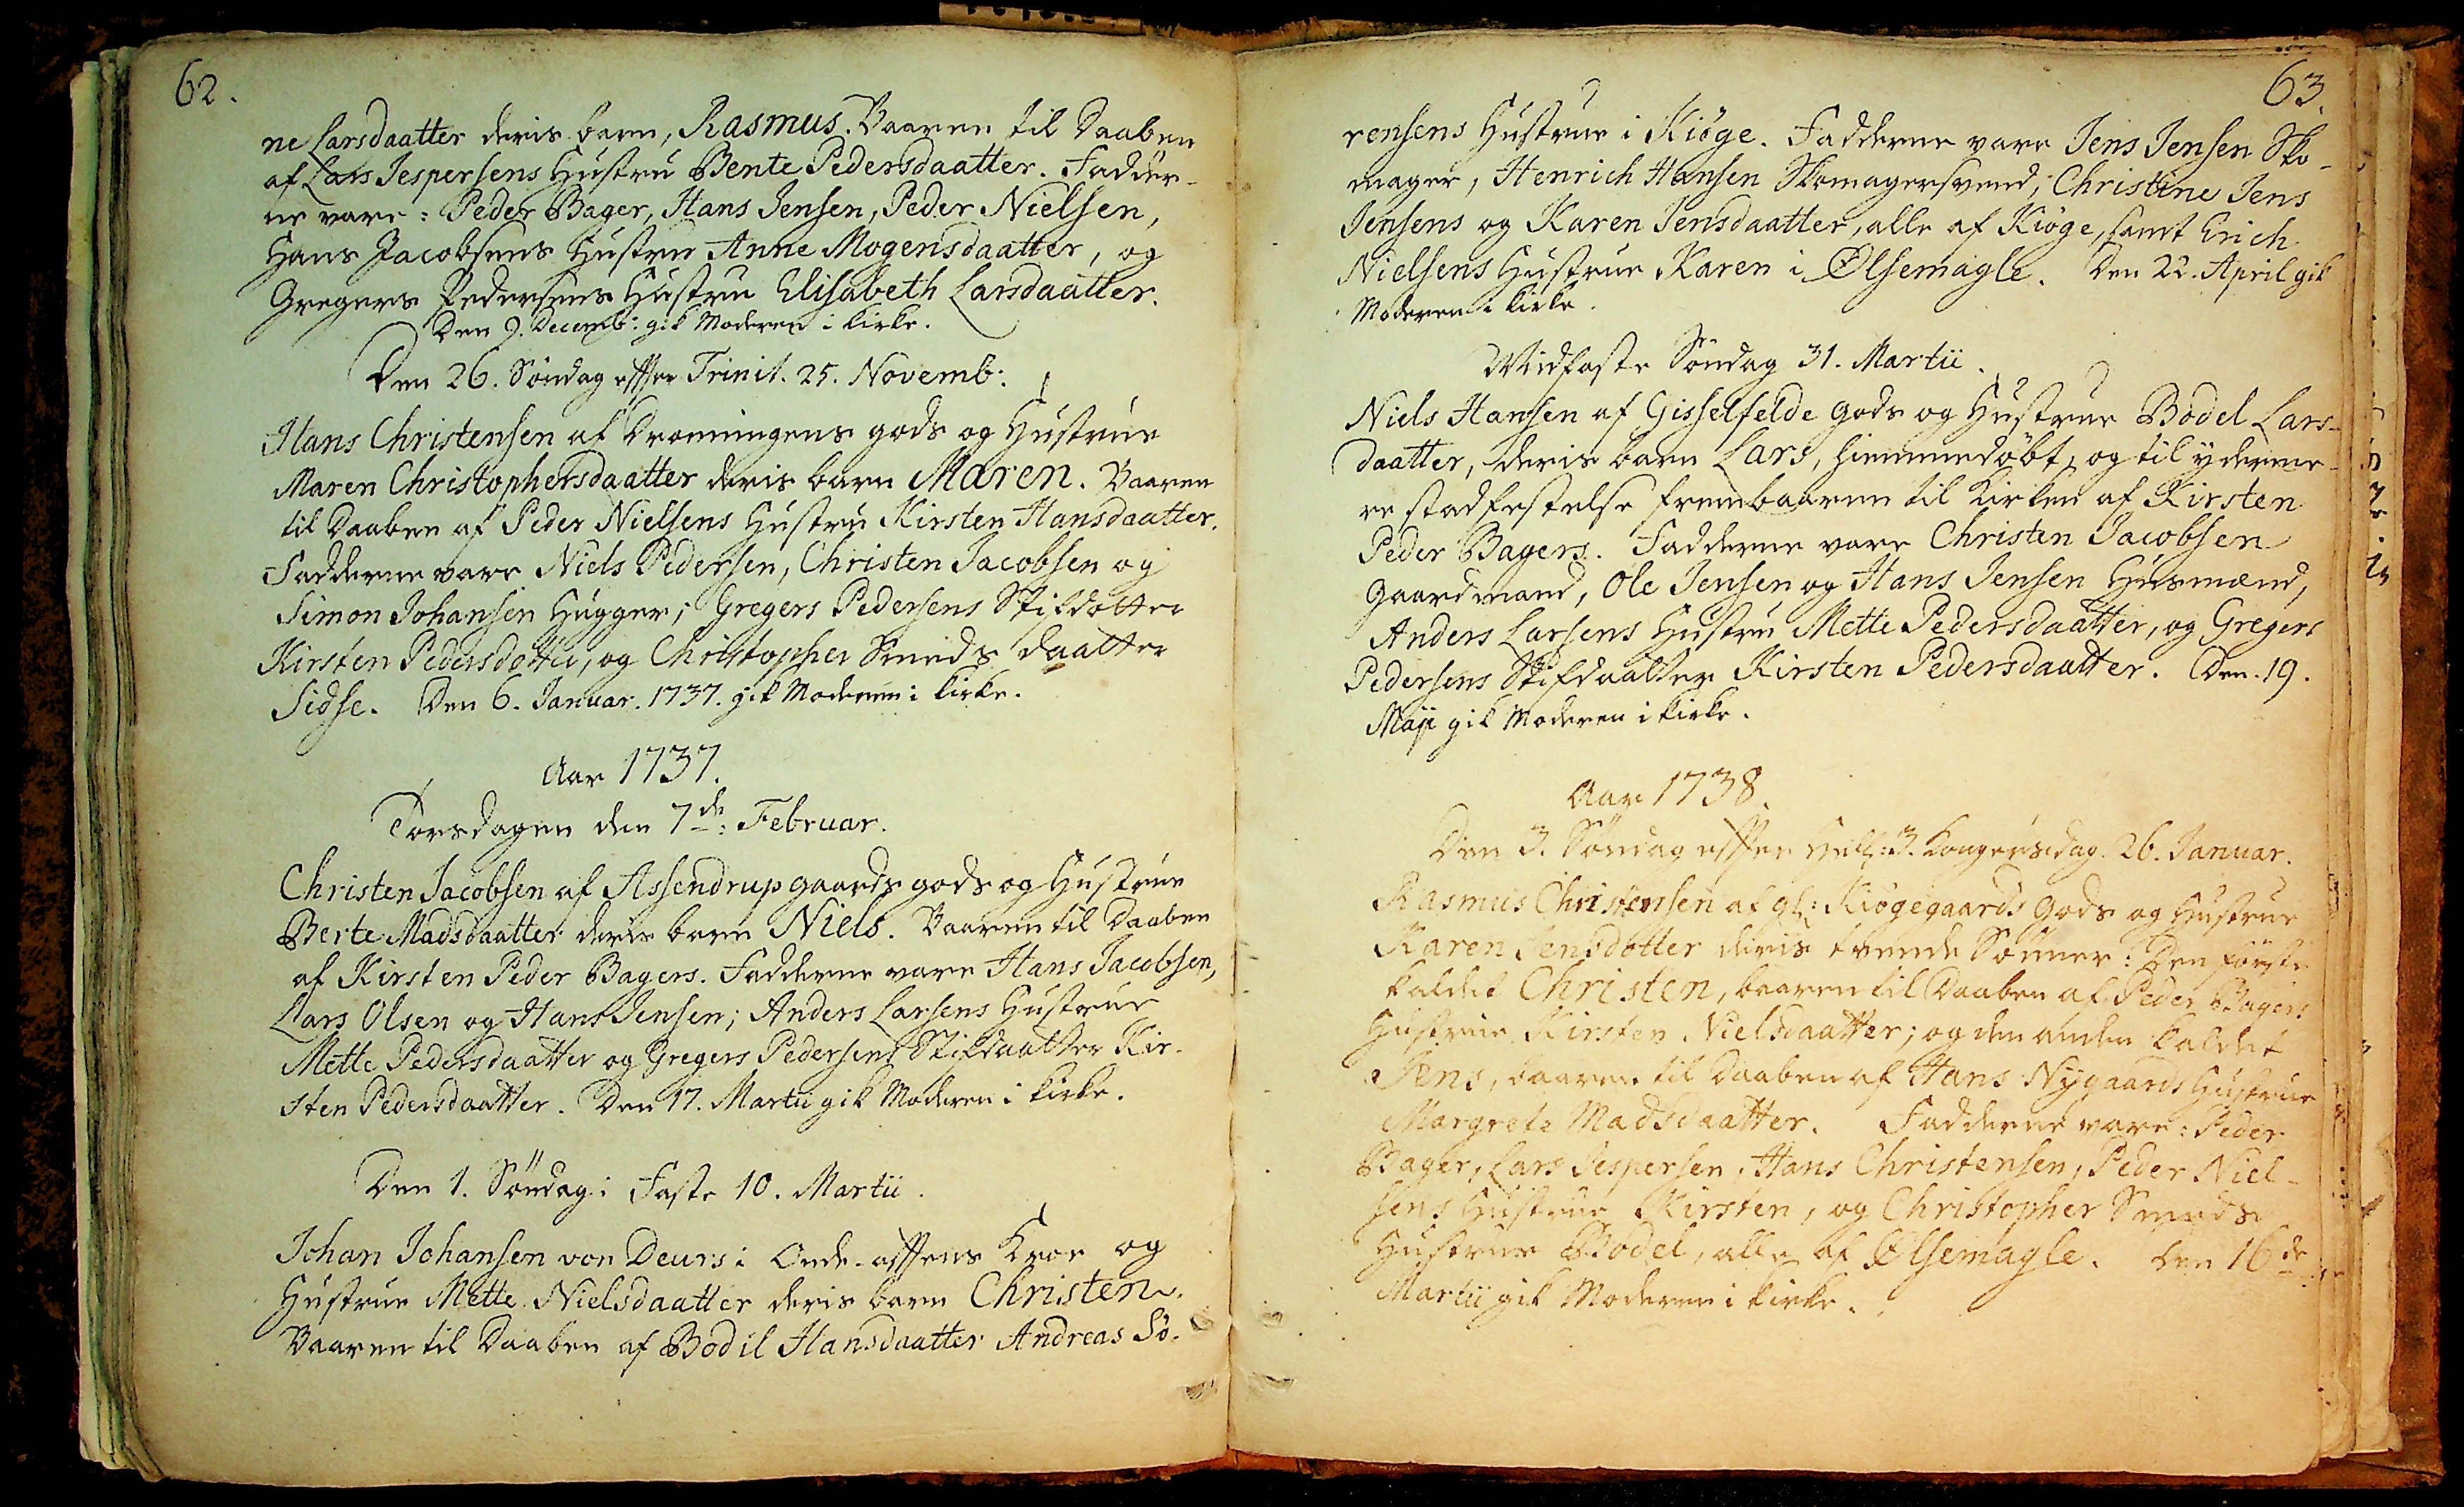62.
ne Larsdaatter deris Barn, Rasmus. Baaren til Daaben
af Lars Jespersens Hustru Bente Pedersdaatter. Fadder¬
ne vare: Peder Bager, Hans Jensen, Peder Nielsen,
Hans Jacobsens Hustrue Anne Mogensdaatter, og
Gregers Pedersens Hustrue Elisabeth Larsdaatter.
Den 9. Decemb: gik Moderen i Kirke
Den 26. Søndag efter Trinit: 25. Novemb:
Hans Christensen af Dronningens Gods og Hustrue
Maren Christophersdaatter deris Barn. Maren. Baaren
til Daaben af Peder Nielsens Hustru Kirsten Hansdaatter.
Fadderne vare Niels Pedersen, Christen Jacobsen og
Simon Johansen Hugger; Gregers Pedersens Stifdatter
Kirsten Pedersdatter, og Christopher Smeds Daatter
Sidse. Den 6. Januar. 1737. gik Moderen i Kirke.
Aar 1737.
Torsdagen den 7de: Februar.
Christen Jacobsen af Assendrup Gaards Gods og Hustrue
Berte Madsdaatter deris Barn Niels. Baaren til Daaben
af Kirsten Peder Bagers. Fadderne vare Hans Jacobsen,
Lars Olsen og Hans Jensen; Anders Larsens Hustrue
Mette Pedersdaatter og Gregers Pedersens Stifdaatter Kir¬
sten Pedersdaatter. Den 17. Martii gik Moderen i Kirke.
Den 1. Søndag i Faste 10. Martii.
Johan Johansen von Deurs i Onde-aftens## Kroe og
Hustrue Mette Nielsdaatter deris Barn Christen.
Baaren til Daaben af Bodil Hansdaatter Andreas Sø¬
63.
rensens Hustrue i Kiøge. Fadderne vare Jens Jensen Sko¬
mager, Henrich Hansen Skomagersvend; Christine Jens
Jensens og Karen Jensdaatter, alle af Kiøge, samt Erich
Nielsens Hustrue Karen i Ølsemagle. Den 22. April gik
Moderen i Kirke.
 Midfaste Søndag 31. Martii
Niels Hansen af Gisselfelde Gods og Hustrue Bodel Lars¬
daatter, deris Barn Lars, hiemmedøbt, og til yderme¬
re stadsfæstelse frembaaren til Kirken af Kirsten
Peder Bagers. Fadderne vare Christen Jacobsen
Gaardmand, Ole Jensen og Hans Jensen Husmænd,
Anders Larsens Hustru Mette Pedersdaatter, og Gregers
Pedersens Stifdaatter Kirsten Pedersdaatter. Den. 19.
Maii gik Moderen i Kirke.
Aar 1738.
Den 3. Søndag efter Hell: 3. Kongersdag. 26. Januar.
Rasmus Christensen af Gl: Kiøgegaards Gods og Hustrue
Karen Jensdaatter, deris tvende Sønner: Den første
kaldet Christen, baaren til Daaben af Peder Bagers
Hustrue Kirsten Nielsdaatter; og den anden kaldet
Jens, baaren til Daaben af Hans Nygaards Hustrue
Margrete Madsdaatter. Fadderne vare: Peder
Bager, Lars Jespersen, Hans Christensen, Peder Niel¬
sens Hustrue Kirsten, og Christopher Smeds
Hustrue Bodel, alle af Ølsemagle. Den 16de
Martii gik Moderen i Kirke. 
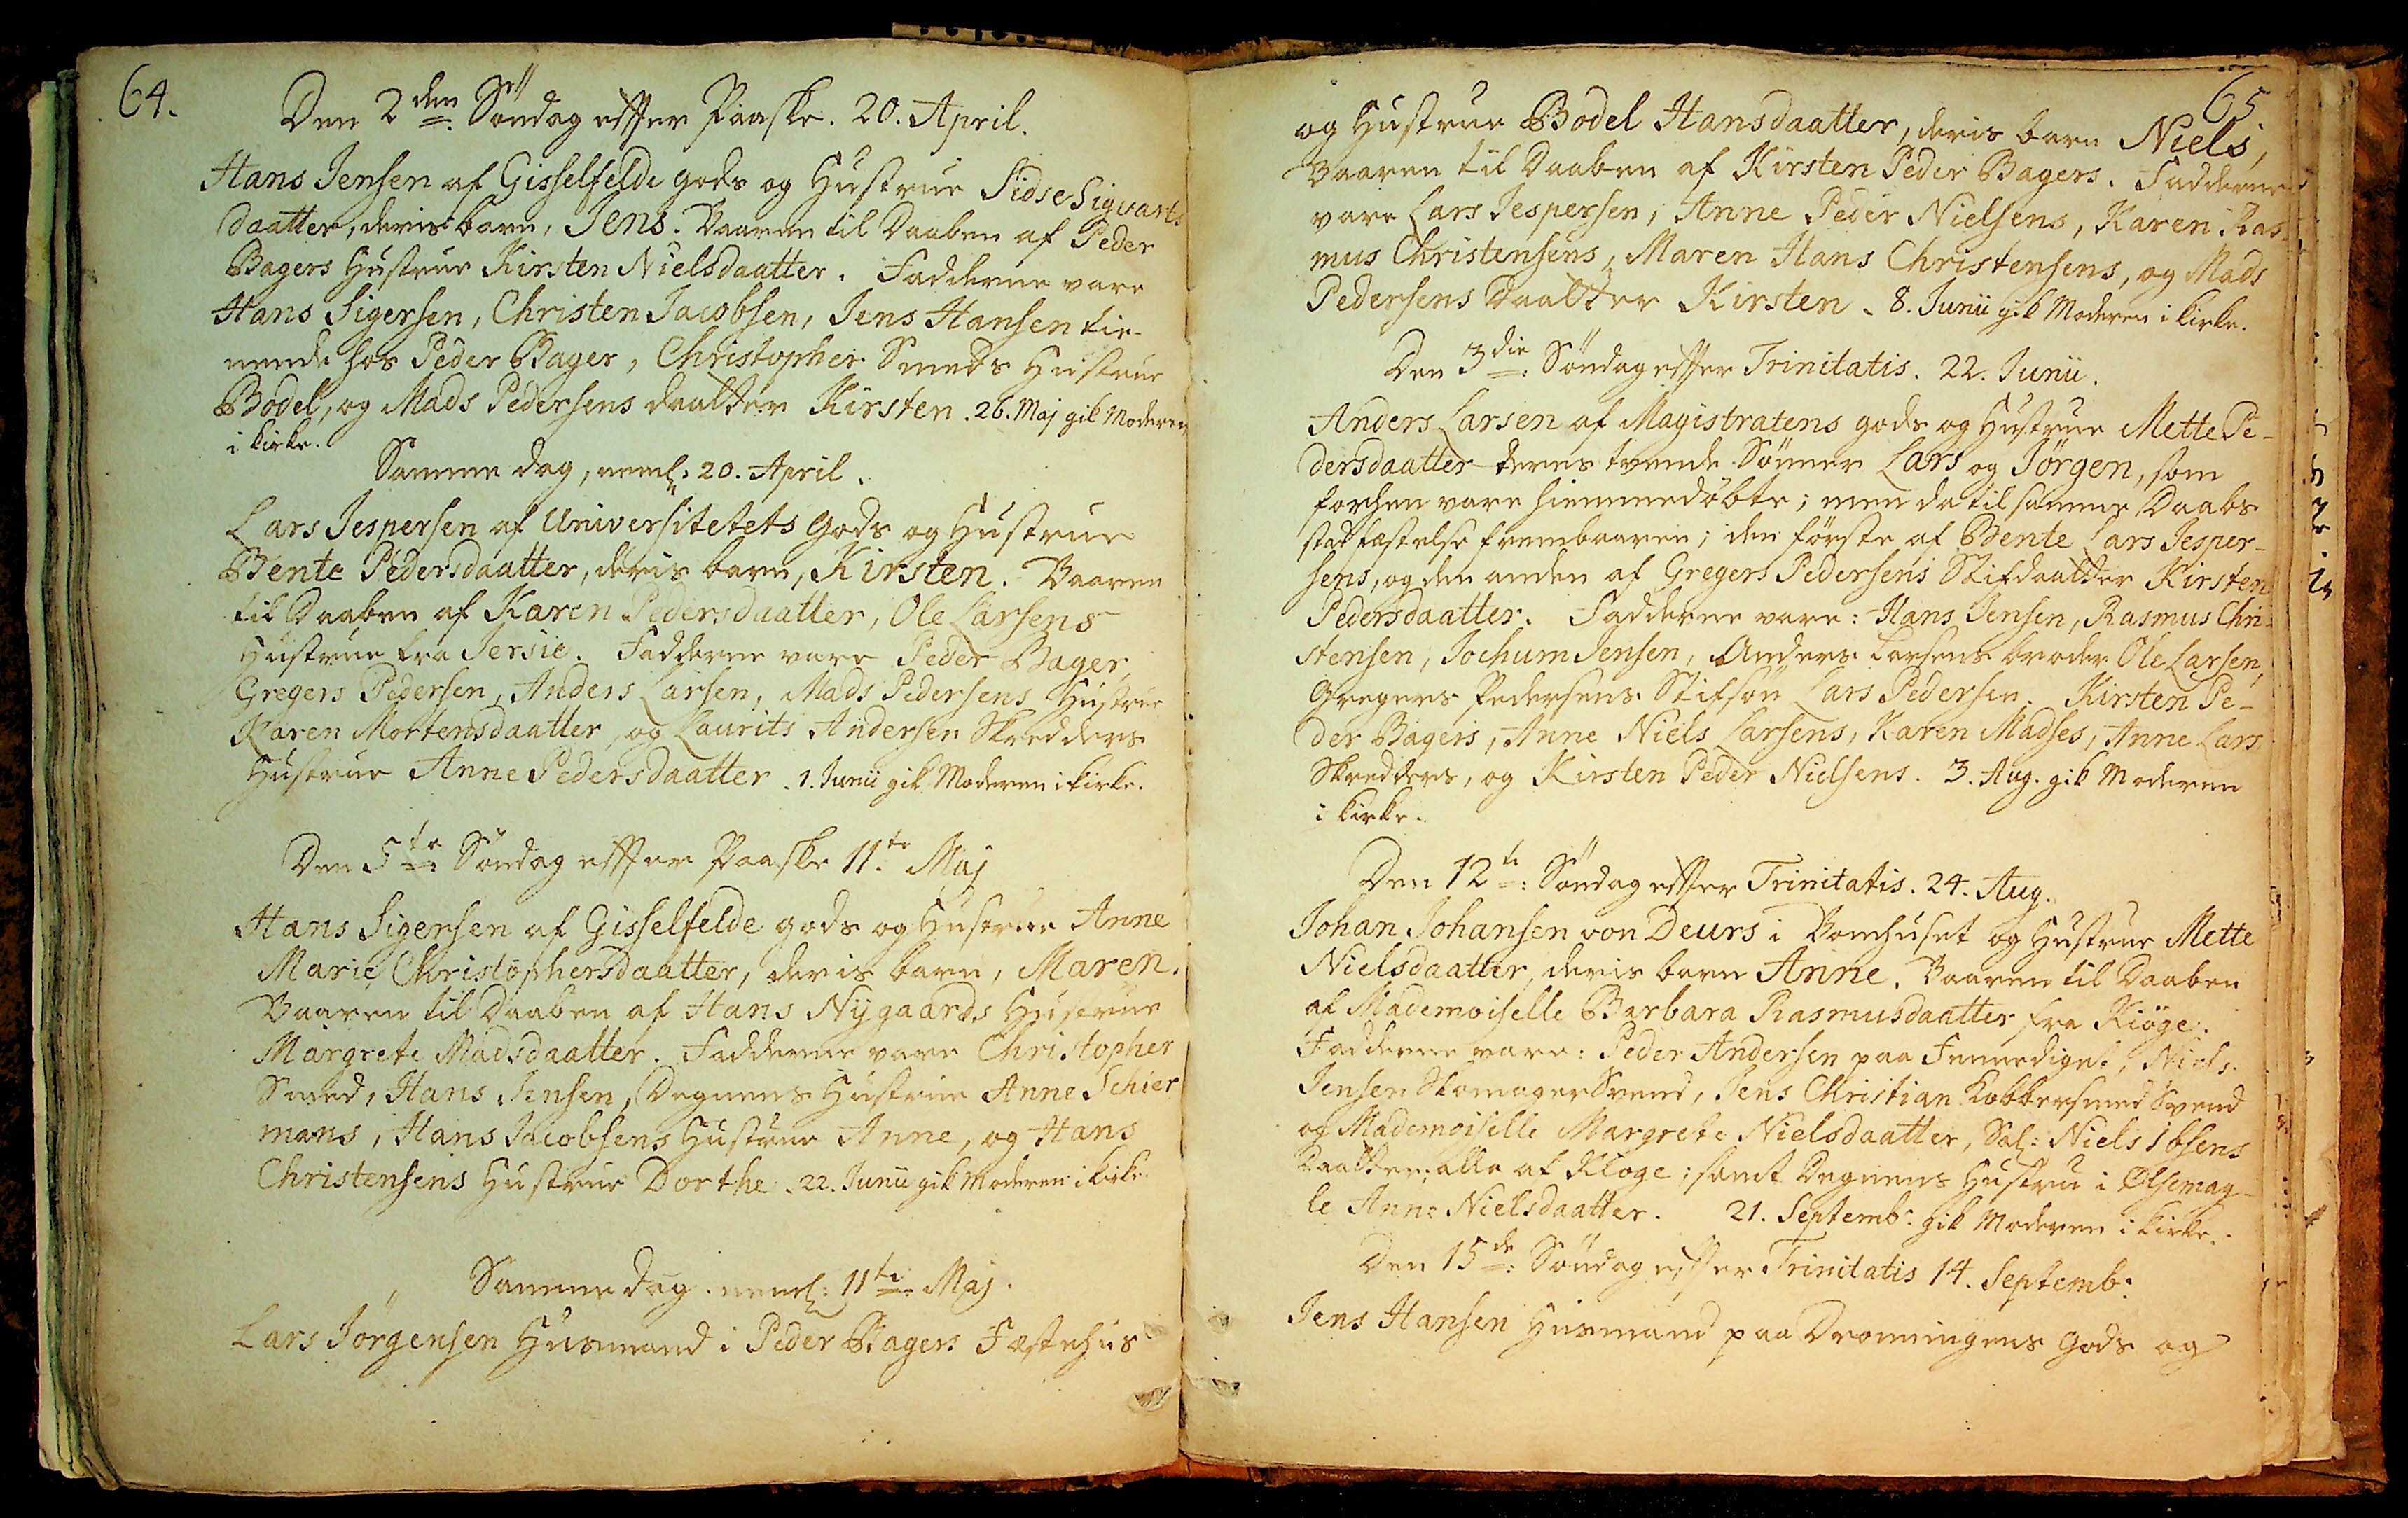64.
Den 2den Søndag efter Paaske. 20. April.
Hans Jensen af Gisselfelde Gods og Hustrue Sidse Sigvarts
daatter, deris Barn, Jens. Baaren til Daaben af Peder
Bagers Hustrue Kirsten Nielsdaatter. Fadderne vare
Hans Sigersen, Christen Jacobsen, Jens Hansen tie¬
nende hos Peder Bager, Christopher Smeds Hustrue
Bodel, og Mads Pedersens daatter Kirsten. 26.Maj gik Moderen
i Kirke.
Samme dag, navnl: 20. April
Lars Jespersen af Universitetets Gods og Hustrue
Bente Pedersdaatter, deris Barn, Kirsten. Baaren
til Daaben af Karen Pedersdaatter, Ole Larsens
Hustrue fra Jersie. Fadderne vare Peder Bager,
Gregers Pedersen, Anders Larsen, Mads Pedersens Hustrue
Karen Mortensdaatter og Laurits Andersen Skredders
Hustrue Anne Pedersdaatter. 1.Junii gik Moderen i Kirke
Den 5te Søndag efter Paaske 11te Maj
Hans Sigersen af Gisselfelde Gods og Hustrue Anne
Marie Christophersdaatter, deris Barn, Maren.
Baaren til Daaben af Hans Nygaards Hustrue
Margrete Madsdaatter. Fadderne vare Christopher
Smed, Hans Jensen, Degnens Hustrue Anne Schier
mans, Hans Jacobsens Hustrue Anne, og Hans
Christensens Hustrue Dorthe. 22.Junii gik Moderen i Kirke.
Samme dag, navnl: 11te Maj
Lars Jørgensen Husmand i Peder Bagers Fæstehus
65.
og Hustrue Bodel Hansdaatter, deris Barn Niels,
Baaren til Daaben af Kirsten Peder Bagers. Fadderne
vare Lars Jespersen, Anne Peder Nielsens, Karen Ras
mus Christensens, Maren Hans Christensens, og Mads
Pedersens Daatter Kirsten. 8.Junii gik Moderen i Kirke.
Den 3die, Søndag efter Trinitatis. 22. Junii.
Anders Larsen af Magistratens Gods og Hustrue Mette Pe¬
dersdaatter deris tvende Sønner Lars og Jørgen, som
forhen vare hiemmedøbte; men da til samme Daabs
stadfæstelse frembaaren; den første af Bente Lars Jesper¬
sens, og den anden af Gregers Pedersens Stifdaatter Kirsten
Pedersdaatter. Fadderne vare: Hans Jensen, Rasmus Chri¬
stensen, Jochum Jensen, Anders Larsens Broder Ole Larsen,
Gregers Pedersens Stifsøn Lars Pedersen. Kirsten Pe¬
der Bagers, Anne Niels Larsens, Karen Madses, Anne Lars
Skredders, og Kirsten Peder Nielsens. 3.Aug. gik Moderen 
i Kirke.
Den 12te Søndag efter Trinitatis. 24. Aug.
Johan Johansen von Deurs i Bomhuset og hustrue Mette
Nielsdaatter, deris Barn Anne. Baaren til Daaben
af Mademoiselle Barbara Rasmusdaatter fra Kiøge.
Fadderne vare: Peder Andersen paa Fennediget, Niels
Jensen Skomager Svend, Jens Christian Kobbersmed Svend
og Mademoiselle Margrete Nielsdaatter, Sal: Niels Ibsens
Daatter; alle af Kiøge; samt Degnens Hustru i Ølsemag¬
le Anne Nielsdaatter. 21.Septemb: gik Moderen i Kirke.
Den 15de: Søndag efter Trinitatis 14. Septembr:
Jens Hansen Husmand paa Dronningens Gods og